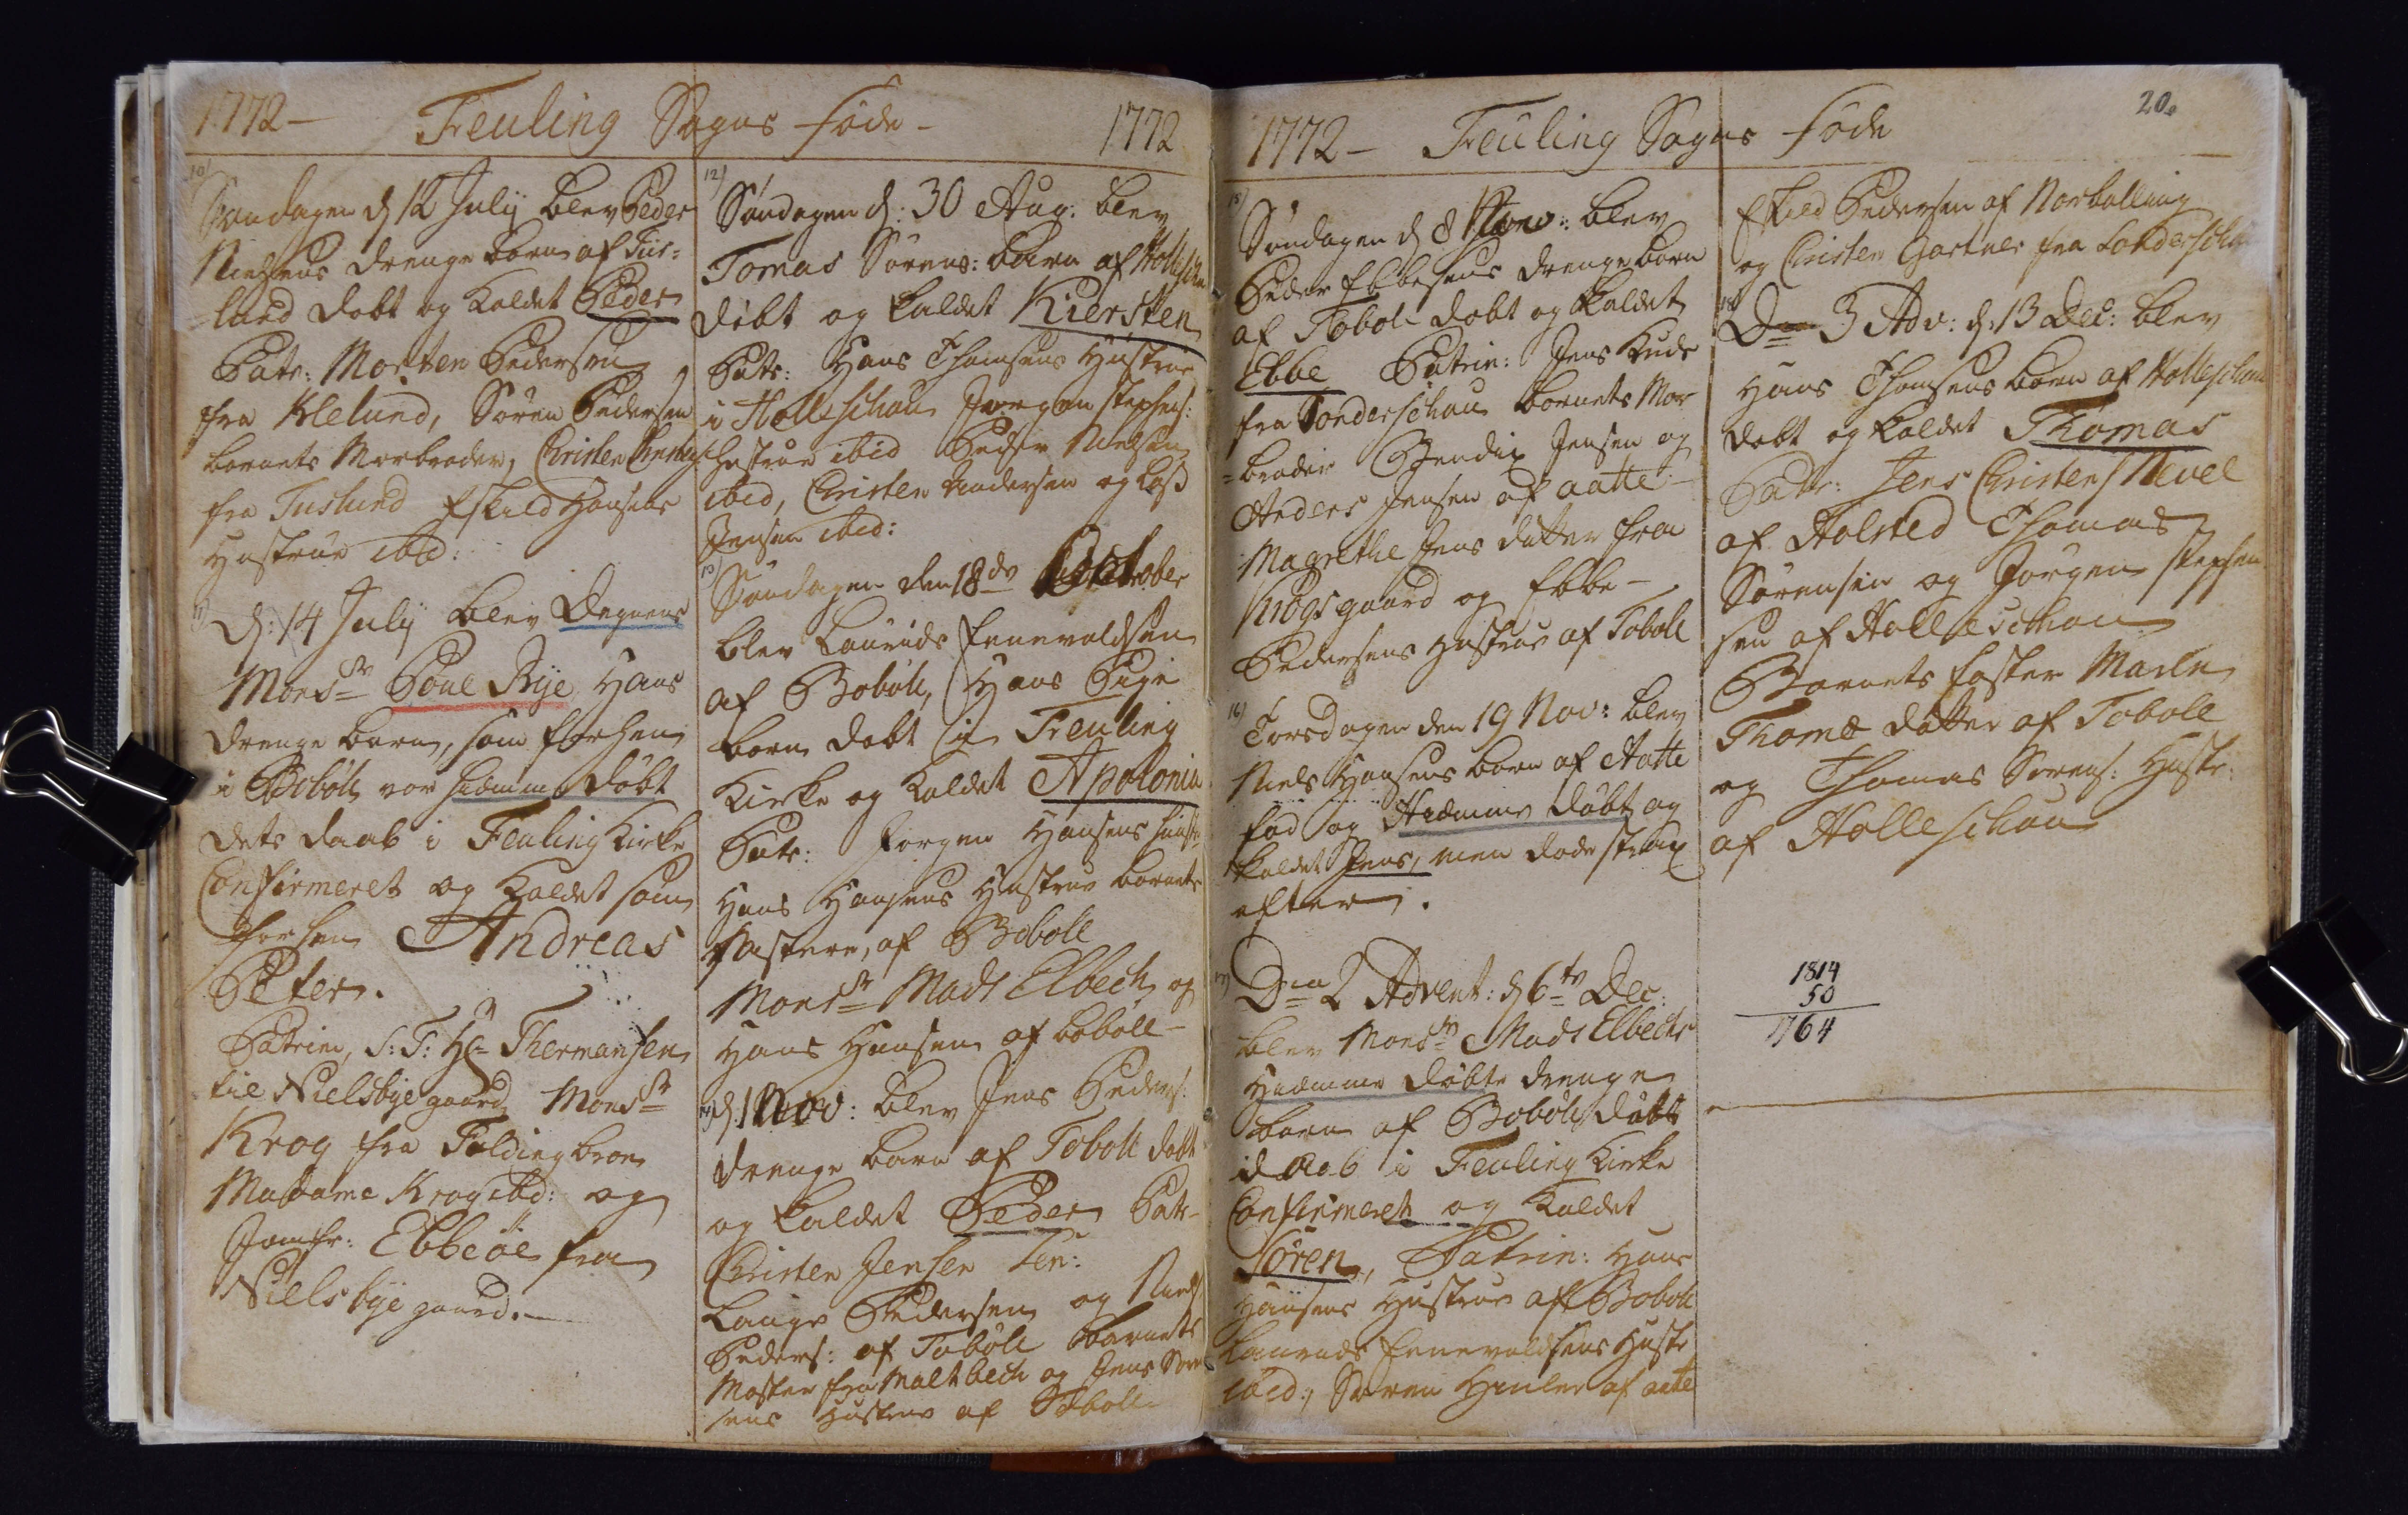1772
Feuling Sogns Føde -
10)
Sondagen d 12 Julij Blev Peder
Nielsens Drenge Barn af Tiis¬
= lundlund dobt og kaldet Peder
Patr: Morten Pedersen
fra Klelund, Søren Pedersens
Barnets Morbroder, Christen Christens
fra Tiislund Eskild Hansens
Hustrue ibid:
11) d: 14 Julij blev Degnens
Monsr Poul Rye, Hans
Drenge Barn, som forhen
i Bobøll var hiemme døbt
dets Daab i Feuling Kirke
Confirmeret og kaldet som
forhen Andreas
Peter
Patrini,, S: T: Hlr Thermansen
til Nielsbye gaard. Monsr
Krog fra Folding broe
Madame Krog ibd: og
Jomfr: Ebbeøe fra
Nielsbye gaard.
1772
12)
Søndagen d: 30 Aug: blev
Tomas Sørens: barn af Holleschou
døbt og kaldet Kiersten
Patr: Hans Thomsens Hustrue
i Holleschou Jorgen stephens:
hustrue ibid: Peder Nielsen
ibid, Christen Andersen og Lass
Jensen ibid:
13)
Søndagen den 18de October
blev Laurids Eenevoldsen
af Bobøll, Hans Pige
barn døbt i Feuling
Kirke og kaldet Apolonia
Patr: Jørgen Hansens Huustr
Hans Hansens Hustrue barnets
##stere af Boboll
Monsr Madz Elbech og
Hans Hansen af Bobøll -
14) d: 1 Nov: Blev Jens Peders:
Drenge Barn af Toboll dobt
og kaldet Peder Patr -
Christen Jensen Jsen:
Lauge Pedersen og Niels
Peders: af Tobøll Barnets
Moster fra Maltbech og Jens Soren
sens Hustrue af Toboll
1772 Feuling Sogns føde
15)
Søndagen d 8 Nov: Blev
Peder Ebbesens Drenge Barn
af Toboll dobt og kaldet
Ebbe Patrin: Jens Knuds
fra Sonderschou Boenets Mor
= Broder, Bendix Jensen og
Anders Jensen af Aatte
Magerethe Jens Datter fra
Krogsgaard og Ebbe
Pedersens Hustrue af Toboll
16)
Torsdagen den 19 Nov: Blev
Niels Hansens Barn af Aatte
fod og Hiæmme døbt og
kaldet Jens, men dode strax
efter
17)
Dca 2 Advent: d 6te Dec:
Blev Monsr Madz Elbechs
Hiæmme døbte drenge.
Barn af Bobøll døbt
Daab i Feuling Kirke
Confirmeret og kaldet
Søren Patrin: Hans
Hansens Hustrue af Boboll
Laurids Eenevoldsens Hustr
ibid: Soren Hiuler af Aatte
20.
Eskild Pedersen af Norbolling
og Christen Gartner fra Sonderschou
18)
Dca 3 Adv: d: 13 Dec: Blev
Hans Thomsens Barn af Holleschou
døbt og kaldet Thomas
Patr:: Jens Christens Nevel
af Holsted Thomas
Sørensen og Jørgen stephan
sen aff Holleschou
Barnets Faster Maren
Thomæs Datter aff Toboll
og Thomas Sørens: Hustr:
af Holleschou
1814
50
1764
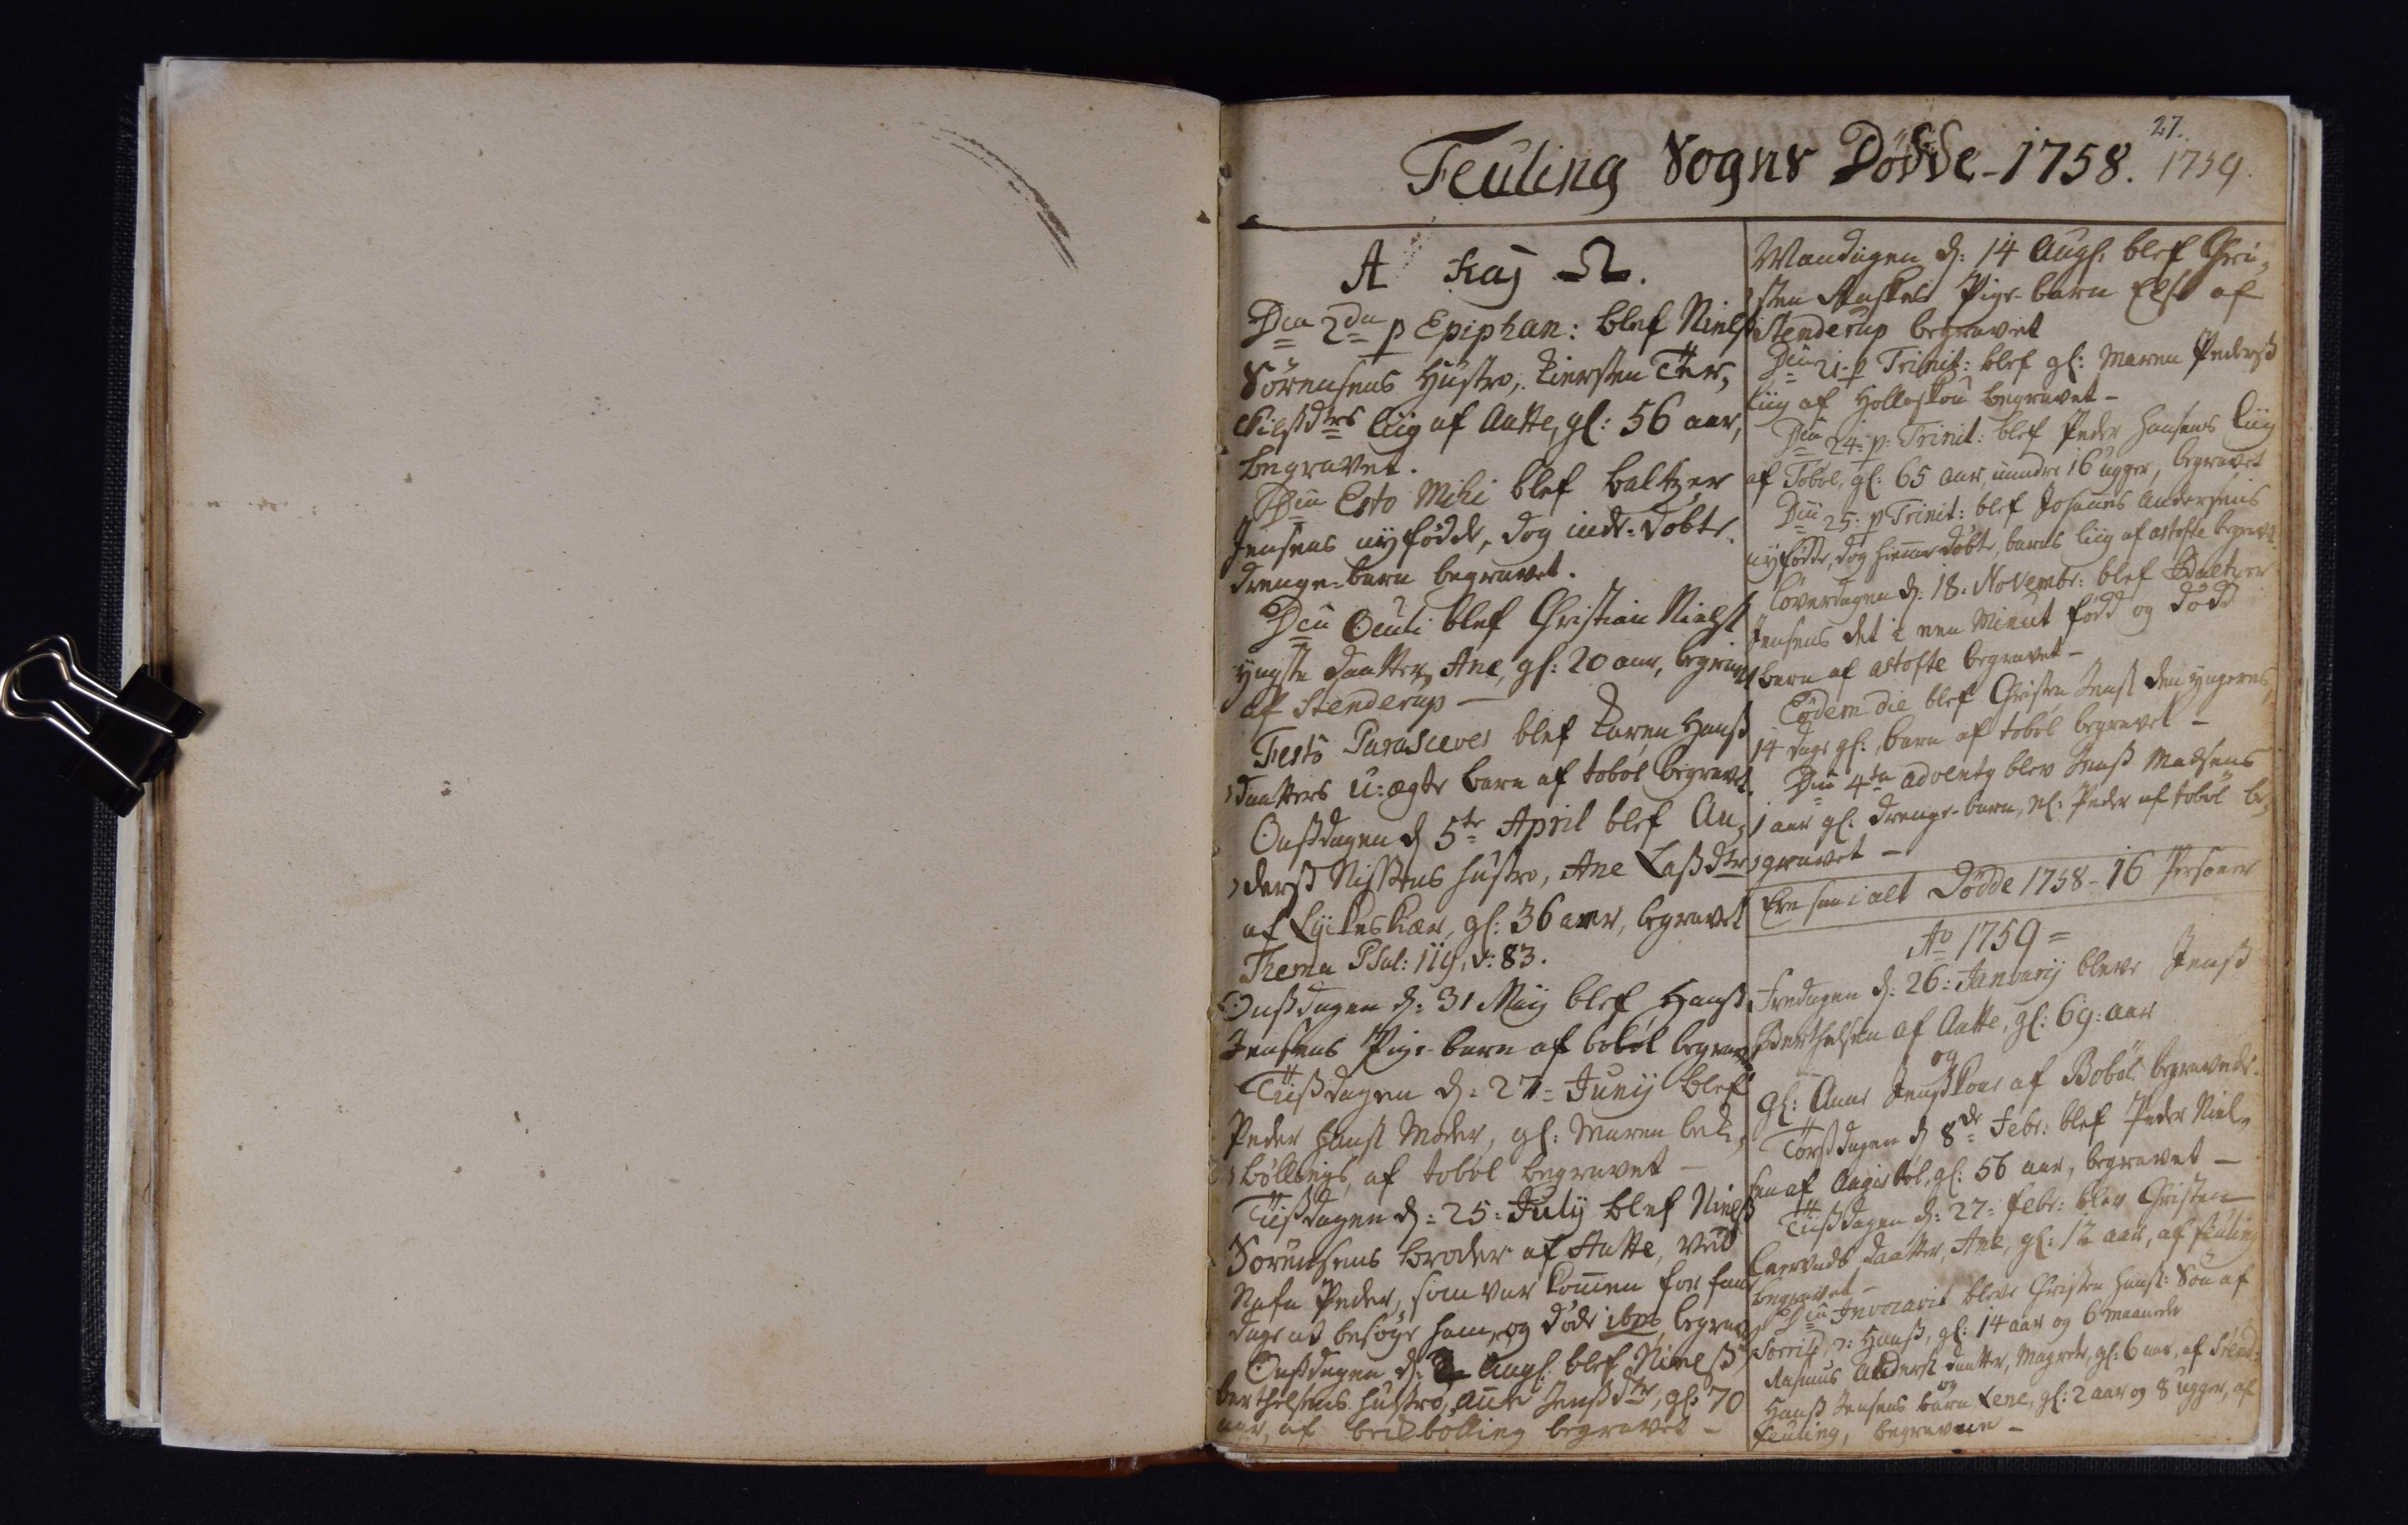Feuling Sogn. Dødde - 1758. 
A Kaj Ω.
Dca 2da p Epiphan: bleff Niels
Sørensens Hustru, Kiersten Ter¬
chilsdtrs liig af Aatte, gl: 56 aar,
begravet.
Dca Esto Mihi bleff Baltzer
Jensens nyfødde, dog inde=døbte
Drengebarn begravet.
Dca Oculi blef Christian Niels
yngste Daatter Ane, gl: 20 Aar, begravet.
af Stenderup
Festo Paradces blef Karen Hans
daatters u:ægte barn af tobøl begraven.
Onsdagen d 5te April bleff An¬
ders Nissens Hustro, Anne Lassdtr
af Lygkeskiær, gl: 36 aar, begravet
Thema Psal: 119,: v: 83.
Onsdagen d: 31 Maij bleff Hans
Jensens Pige-barn af bobøl begravet
Tiisdagen d: 27 Junij blef
Peder Hans Moder, gl: Maren bek¬
bøllings af tobøl begravet
Tiisdagen d: 25: Julij bleff Niels
Sørensens Broder af Aatte, ved
Nafn Peder, som var kommen for faa
dage at besøge ham, og døde ibm begravet
Onsdagen d: 2 Augs: bleff Nielss
Berthelsens Hustro, Aude Jenssdtr, gl: 70
aar af beckbolling begravet
27.
1759
Mandagen d: 14 Augs: blef Chri¬
sten Raskes Pige-barn Else af
Stenderup begravet
Dca 21 p Trinit: blef gl: Maren Pederss
liig af Holleskou begravet -
Dca 24: p: Trinit: blef Peder Hansens liig
af Tobøl, gl: 65 Aar, ,mindre 16 ugger, begravet
Dca 25: p Trinit: blef Johannes Andersens
nyfødde og hiemme døbt barns liig af astofte begravet.
Løverdagen d: 18. Novembr: blef Baltzer
Jensens det i een Minut fødd og dødd
Barn af astofte begravet -
Eodem Die blef Christen Jens den Yngere
14 dage gl: barn af tobøl begravet -
Dca 4 ta Adventy blev Hans Madsens
1 aar gl. Drenge-barn, nl: Peder af tobøl br¬
= gravet
Ere saa i alt Dødde 1758 - 16 Personer
Ao 1759 =
Fredagen d: 26: Januarij bleve Jenss
Bertelsen af Aatte, gl: 69 Aar.
og
gl: Anne Jenss Kone Bobøl begravede.
Torsdagen d 8de Febr: blef Peder Niel¬
sen af Aagisbøl, gl: 56 Aar, begrave -.
Tiissdagen d: 27: Febr: blev Christen
Leervads Daatter, Ane gl: 12 aar, af Feuling
begravet
Dca Invocavit bleve Christen Hanss: Søn af
Sorrild, ɔ: Hanss, gl: 14 Aar og 6 Maaneder
Rasmus Anderss daatter, Magrete, gl: 6 Aar, af tend:
og
Hanss Jensens barn Lene, gl: 2 Aar og 8 ugger, af
Feuling, begravne